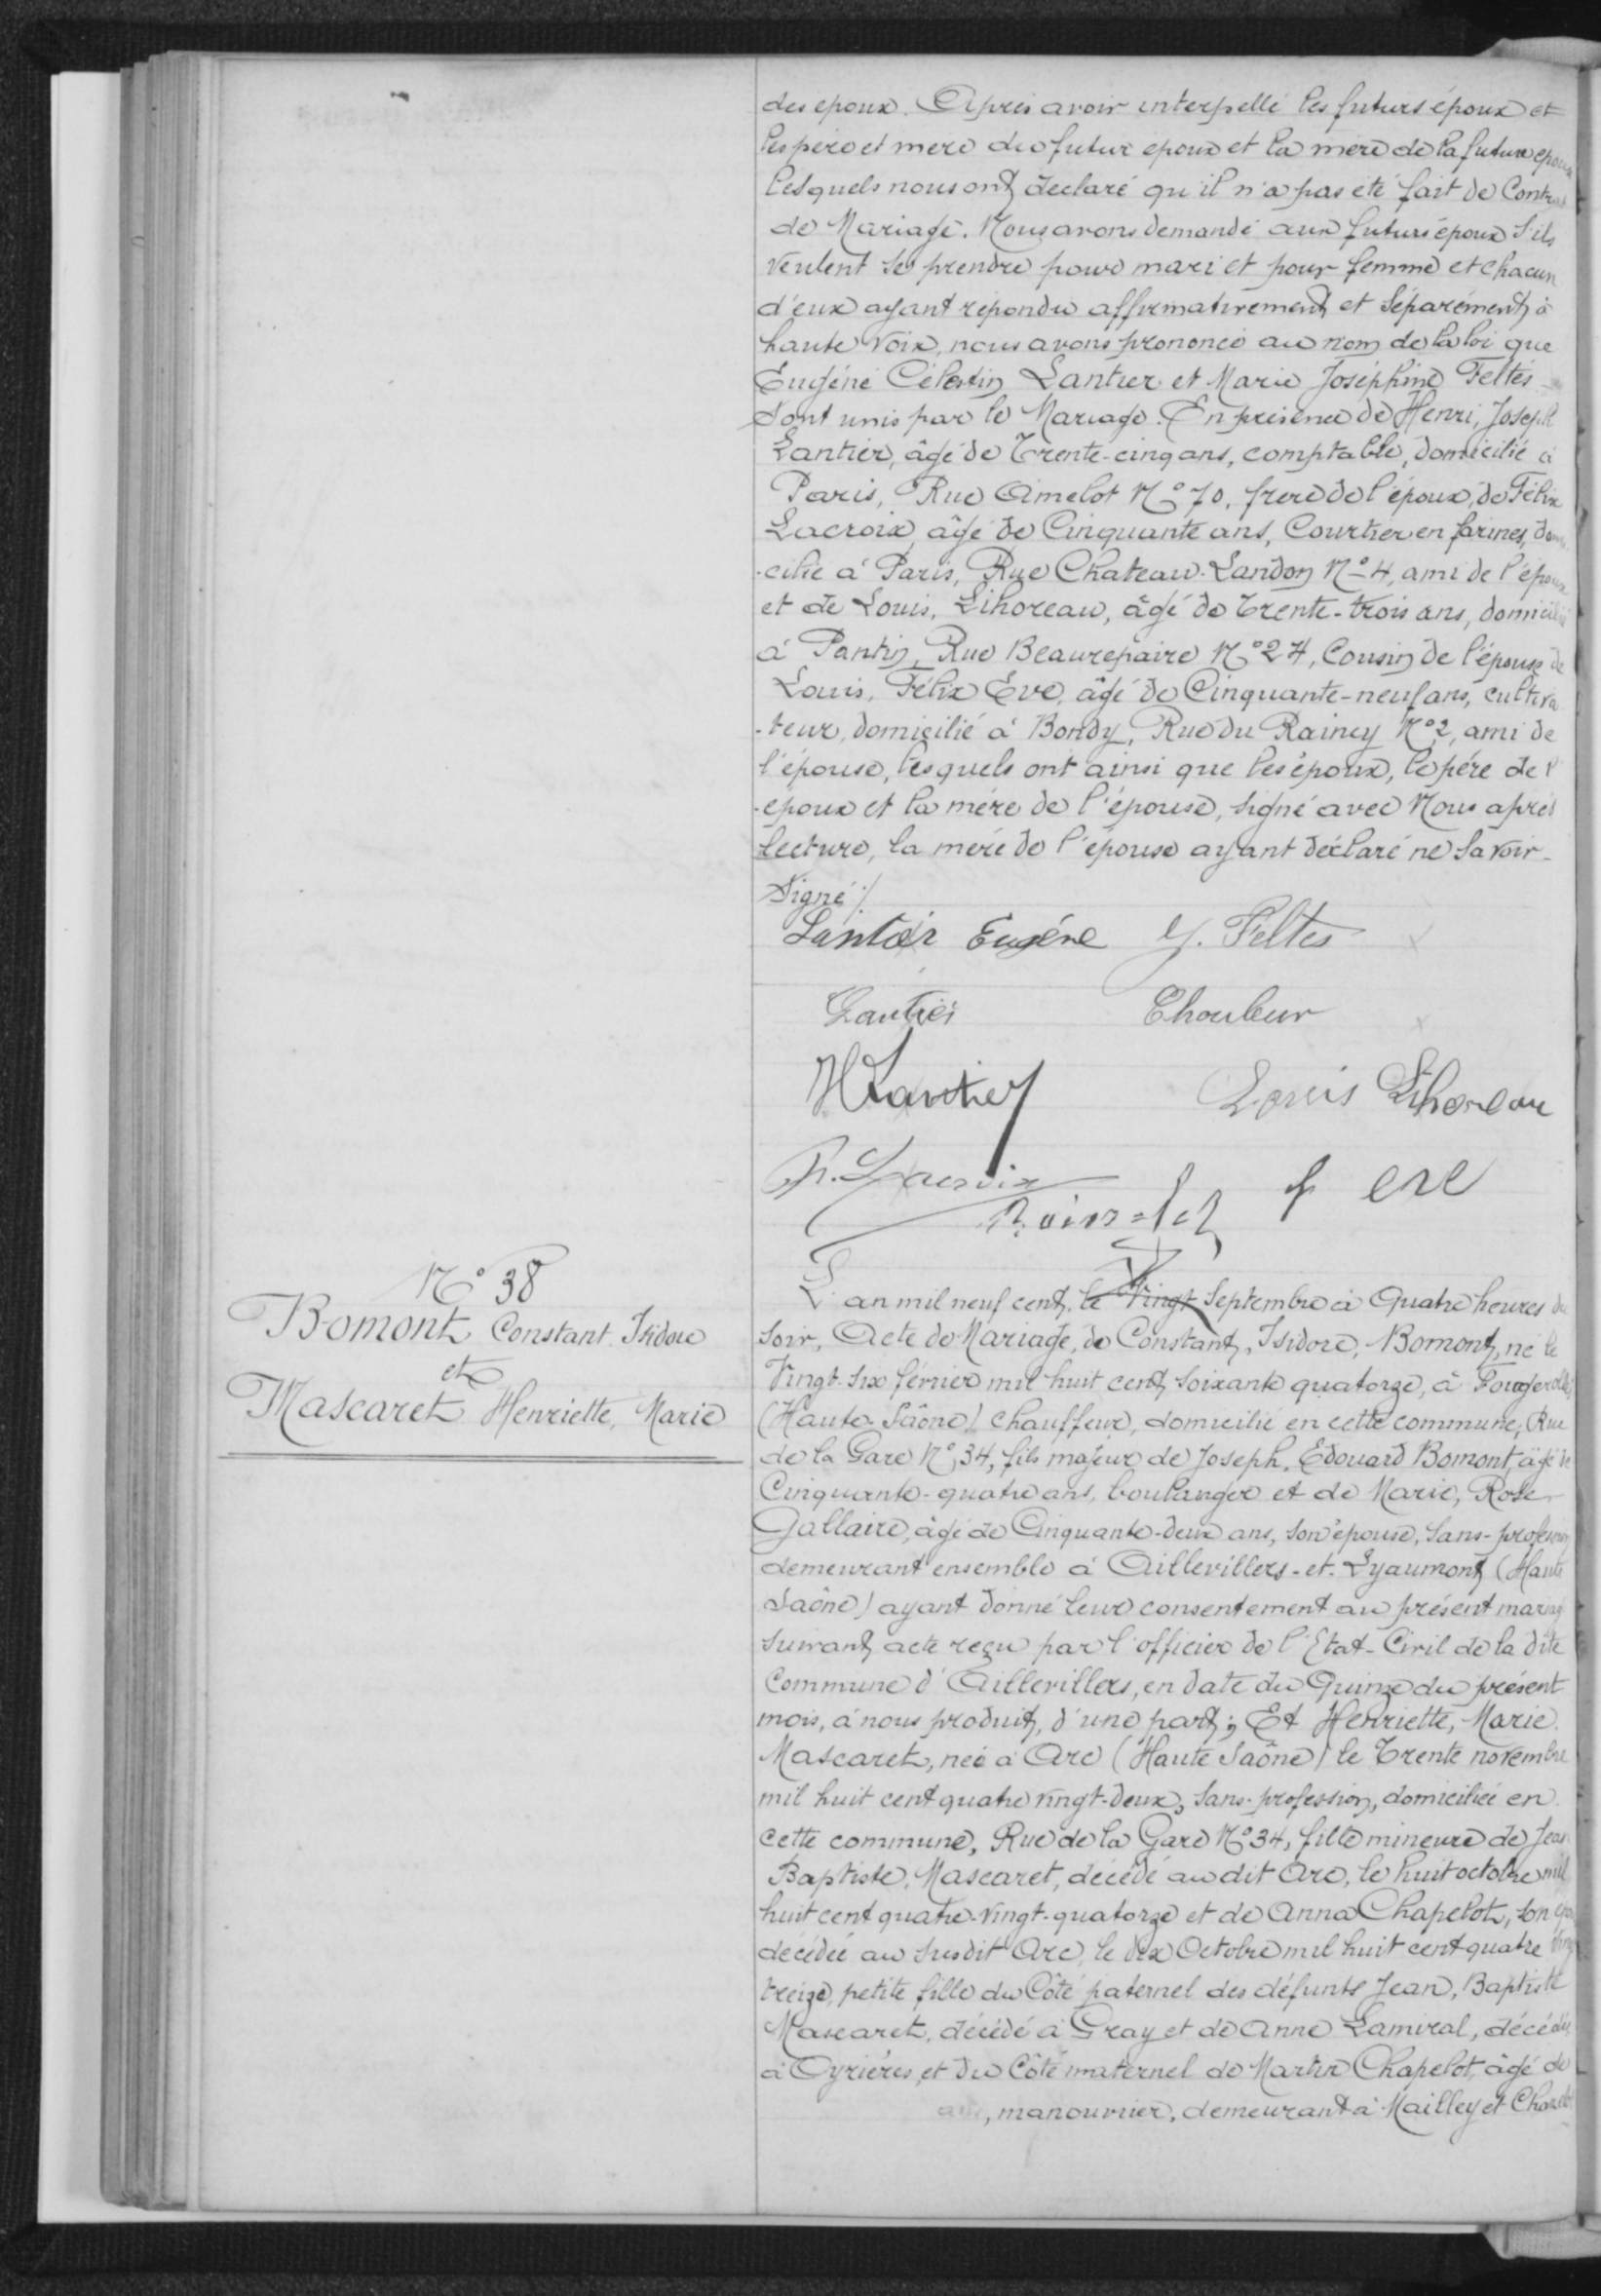des epoux. Après avoir interpellé les futurs époux et
les père et mère du futur epoux et la mere de la future epouse
lesquels nous ont declaré qu'il n'a pas été fait de contrat
de mariage. Nous avons demandé aux futurs époux s'ils
veulent se prendre pour mari et pour femme et chacun
d'eux ayant répondu affirmativement et séparément à
haute voix nous avons prononcé au nom de la loi que
Eugénie Célestin Santier et Marie Joséphine Feltes
sont unis par le Mariage. En présence de Henri, Joseph
Santier, âgé de trente-cinq ans, comptable, domicilié à
Paris, Rue Amelot N°17, frere de l'époux de Félix
Lacroix âgé de cinquante ans, courtier en farines domi
cilié à Paris, Rue Chateau Landon N°4 ami de l'époux
et de Louis, Lihorceau, âgé de trente-trois ans, domicilié
à Pantin, Rue Beaurepaire N°24, Cousin de l'épouse de
Louis, Félix Eve, âgé de cinquante-neuf ans, cultiva
-teur, domicilié à Bondy, Rue du Raincy N°2, ami de
l'épouse, lesquels ont ainsi que les époux, le père de l'
epoux et la mère de l'épouse, signé avec Nous après
lecture, la mère de l'épouse ayant déclaré ne savoir
signée ./.
N°38
Bomont Constant Isidore
et
Mascaret Henriette Marie
L'an mil neuf cent, le Vingt Septembre à quatre heures du
soir, acte de Mariage, de Constant, Isidore, Bomont, né le
vingt-six février mil huit cent soixante quatorze, à Lougerville
(Haute Saône) chauffeur, domicilié en cette commune; Rue
de la Gare N°34, fils majeur de Joseph, Edouard Bomont, âgé de
cinquante-quatre ans, boulanger et de Marie, Rose,
Gallaire, âgé de cinquante-deux ans, son épouse, sans-profession
demeurant ensemble à Aillevillers-et-Lyaumont -Haute
Saône) ayant donné leur consentement au présent maruage
suivante acte reçu par l'officier de l'Etat-Civil de ladite
Commune d'Aillerviller, en date du quinze du présent
mois, à nous produit, d'une part; Et Henriette, Marie
Mascaret, née à Arc (Haute Saône) le Trente novembre
mil huit cent quatre vingt-deux, sans profession, domiciliée en
cette commune, Rue de la Gare N°34, fille mineure de Jean
Baptiste, Mascaret, décédé audit Aro, le huit octobre mil
huit cent quatre vingt quatorze et de Anna Chapelot, son epou
décédée au susdit Arc, le dix Octobre mil huit cent quatre vingt
treize, petite fille du Côté paternel des défunts Jean, Baptiste
Mascaret, décédé à Gray et de Anne Lamiral décédée
à Oyrières, et du Côté maternel de Martin Chapelot, âgé du
manouvrier, demeurant à Mailley et Charet
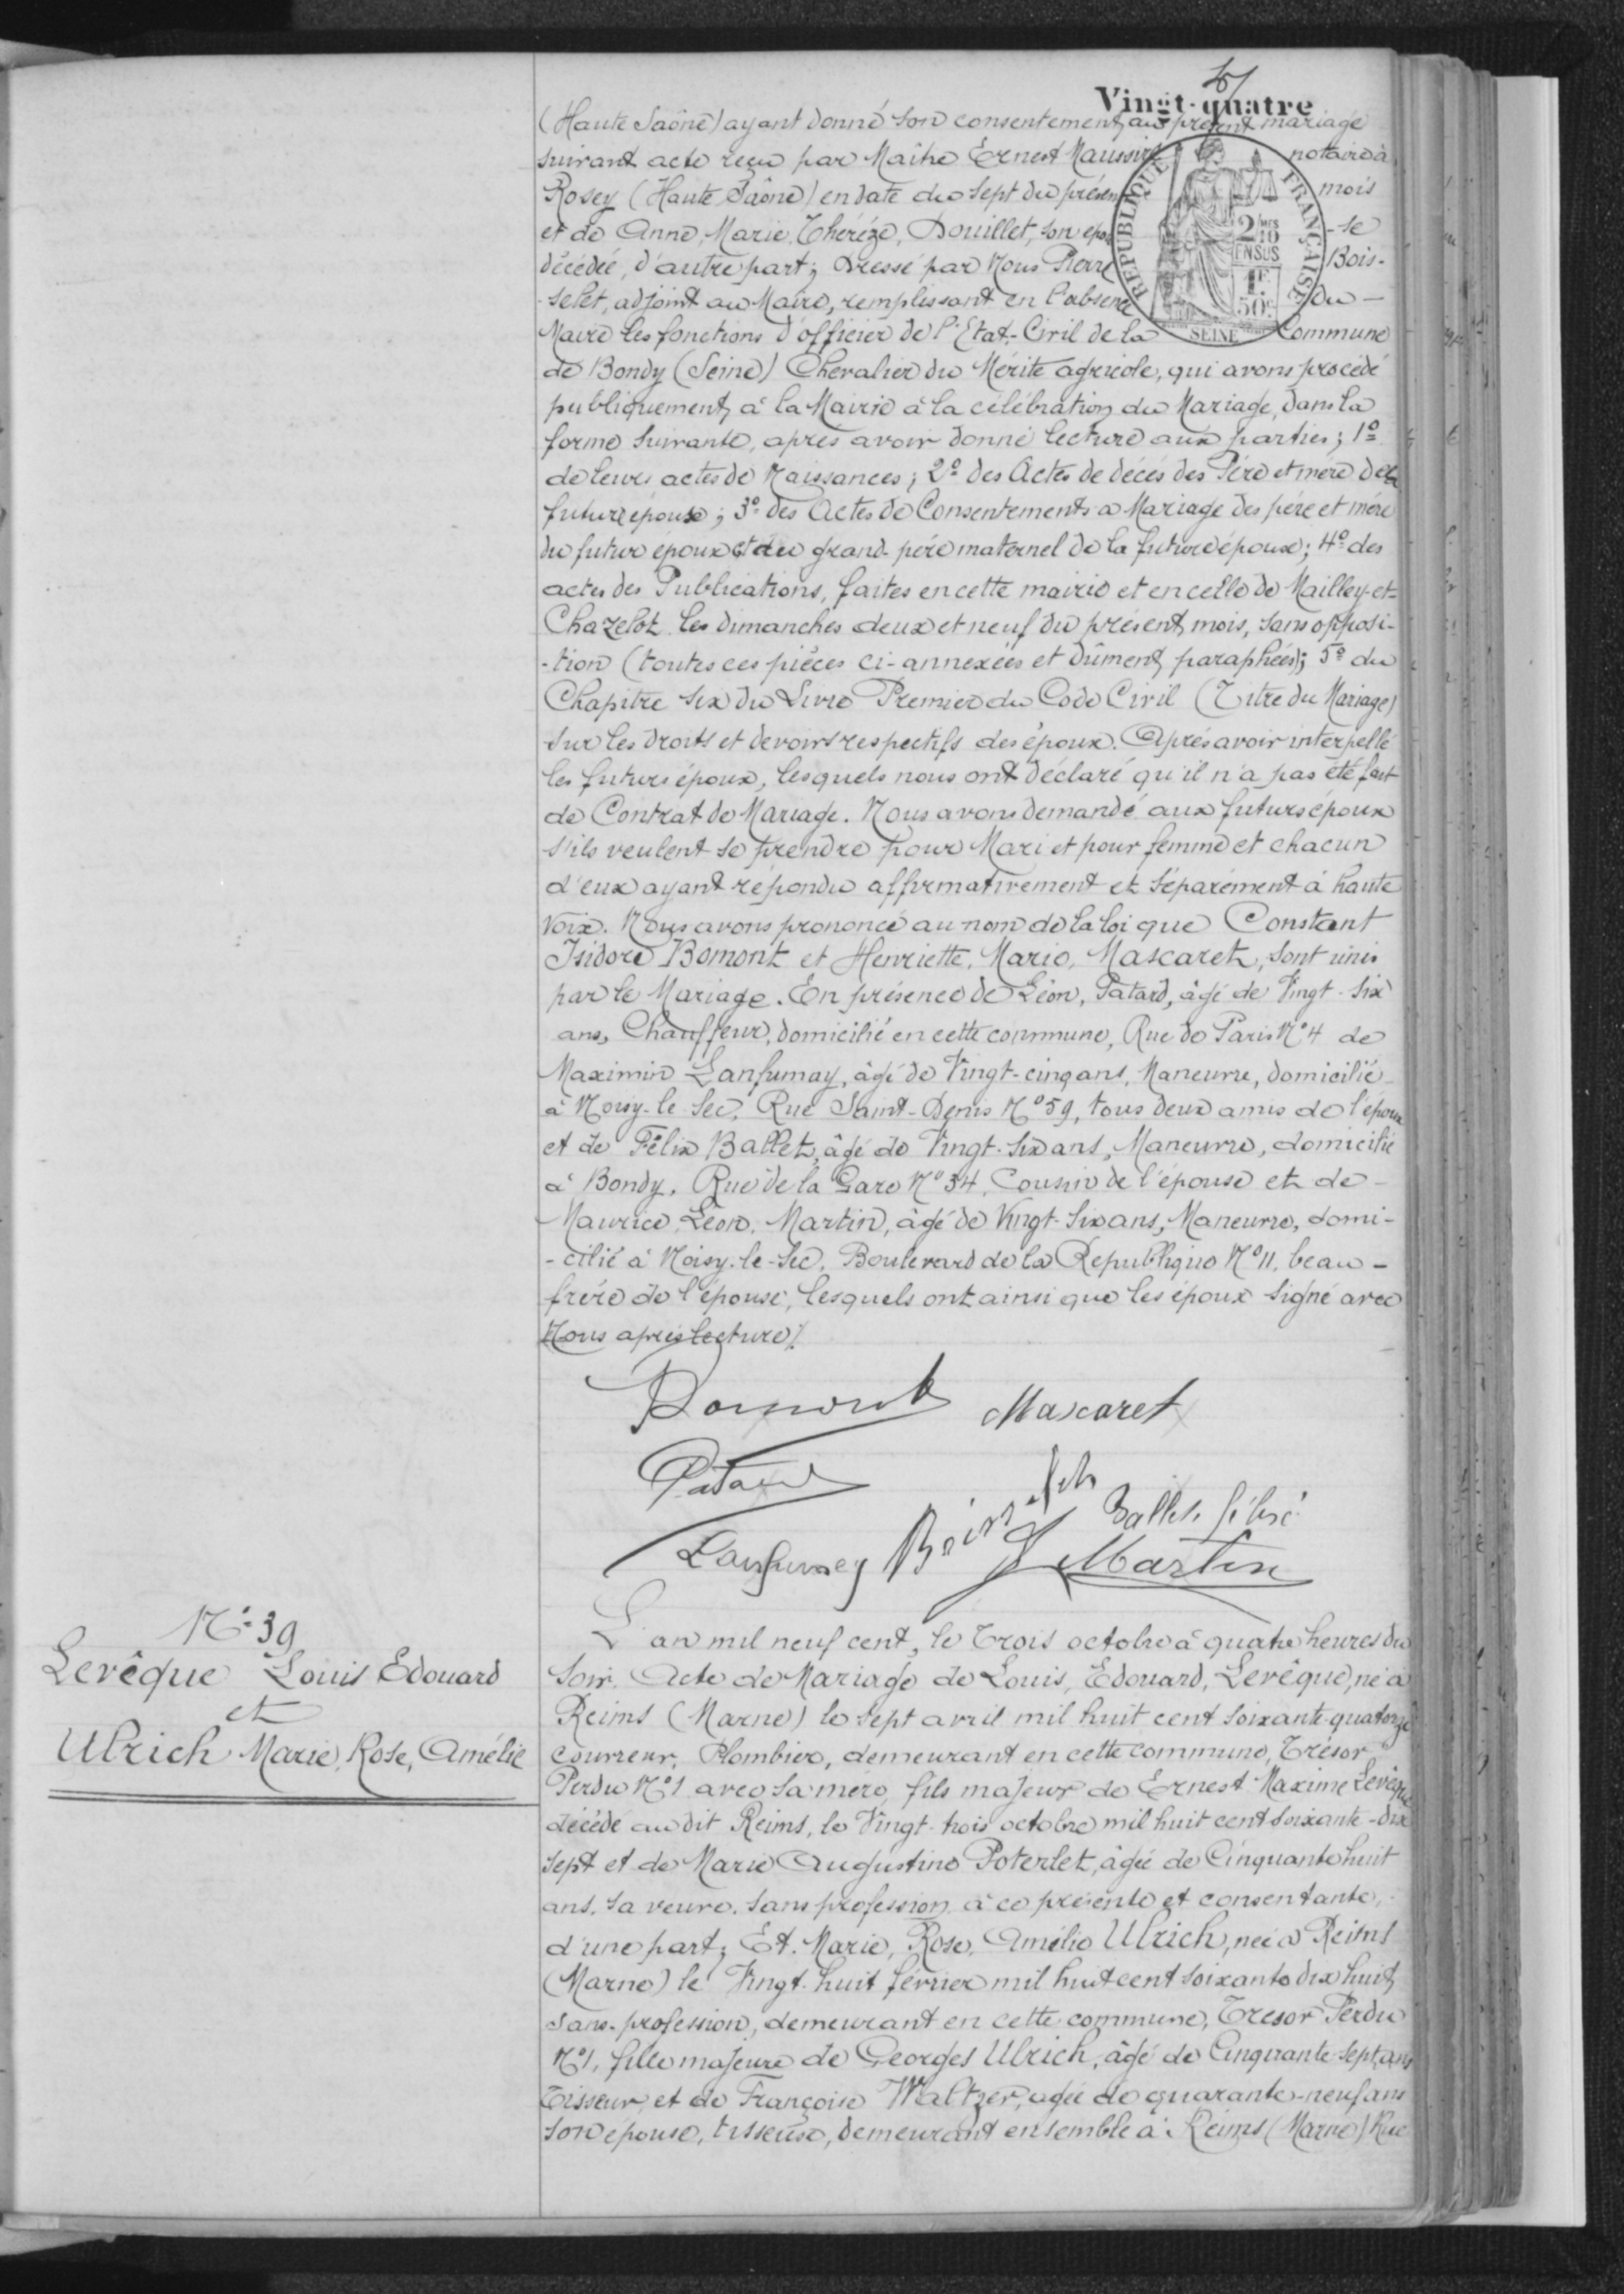(Haute Saône) ayant donné son consentement au présent mariage
suivant acte reçu par Maître Ernest Maussire notaire à
Rosey (Haute Saône) en date des sept du présent mois
et de Anne, Marie, Thérèze, Douillet, son epou-se
décédée, d'autre part; Dressé par Nous Pierre Bois-
selet, adjoint au Maire, remplissant en l'absence du-
Maire les fonctions d'officier de l'Etat Civil de la Commune
de Bondy (Seine) Chevalier du Mérite agricole, qui avons procédé
publiquement à la Mairie à la célébration du Mariage, dans la
forme suivante après avoir donné lecture aux parties; 1°:
de leurs actes de naissances; 2°: des acte de décès des Père et mère de la
future épouse, 3°/ des actes de consentement a Mariage des père et mère
du futur époux et du grand père maternel de la future époux; 4°: des
actes des Publications, faites en cette mairie et en celle de Mailley-et-
Chazelot les dimanches deux et neuf du présent mois, sans opposi-
tion (toutes ces pièces ci-annexées et dûment paraphées); 5°/ du
Chapitre six du Livre Premier du Code Civil (Titre du Mariage)
sur les droits et devoirs respectifs des époux. Après avoir interpellé
les futurs époux, lesquels nous ont déclaré qu'il n'a pas été fait
de Contrat de Mariage. Nous avons demandé aux futurs époux
s'ils veulent se prendre pour Mari et pour femme et chacun
d'eux ayant répondu affirmativement et séparément à haute
voix. Nous avons prononcé au nom de la loi que Constant
Isidore Romont et Henriette Marie Mascaret sont unis
par le Mariage. En présence de Léon, Patard, âgé de Vingt-six
ans, Chauffeur, domicilié en cette commune, Rue de Paris N°4 de
Maximin Lanfumay, âgé de Vingt-cinq ans, maneuvre, domicilié
à Noisyèle-Sec, Rue Saint-Denis N°59, tous deux amis de l'époux
et de Félix Ballet, âgé de vingt-six ans, Maneuvre, domicilié
à Bondy, Rue de la Gare N°34, Cousin de l'épouse et de
Maurice, Léon, Martin, âgé de vingt-six ans, Maneuvre, domi-
-cilié à Noisy-le-Sec, Boulevard de la République N°11, beau-
frère de l'épouse, lesquels ont ainsi que ls époux signé avce
Nous après lecture ./.
N°39
Levêque Louis Edouard
et
Ulrich Marie Rose Amélie
L'an mil neuf cent le Trois octobre à quatre heures du
soir. Acte de Mariage de Louis, Edouard, Levêque né à
Reims (Marne) le sept avril mil huit cent soixante quatorze
couvreur, pombier, demeurant en cette commune, Trésor
Perdu N°1 avec sa mère, fils majeur de Ernest Maxime Levêque
décédé audit Reims, les Vingt-trois octobre mil huit cent soixante-dix
sept et de Marie Augustine Portelet, âgée de cinquante huit
ans, sa veuve, sans profession, à ce présente et consentante
d'une part; Et Marie, Rose, Amélie Ulrich, née à Reims
(Marne) le Vingt huit février mil huit cent soixante-dix-huit
sans profession, demeurant en cette commune, Tresor Perdu
N°1, fille majeure de Georges Ulrich, âgé de cinquante sept ans
tisseur, et de Françoise Walter, agée de quarante-neuf ans
son épouse, tisseuse, demeurant ensemble à Reims (Marne) Rue